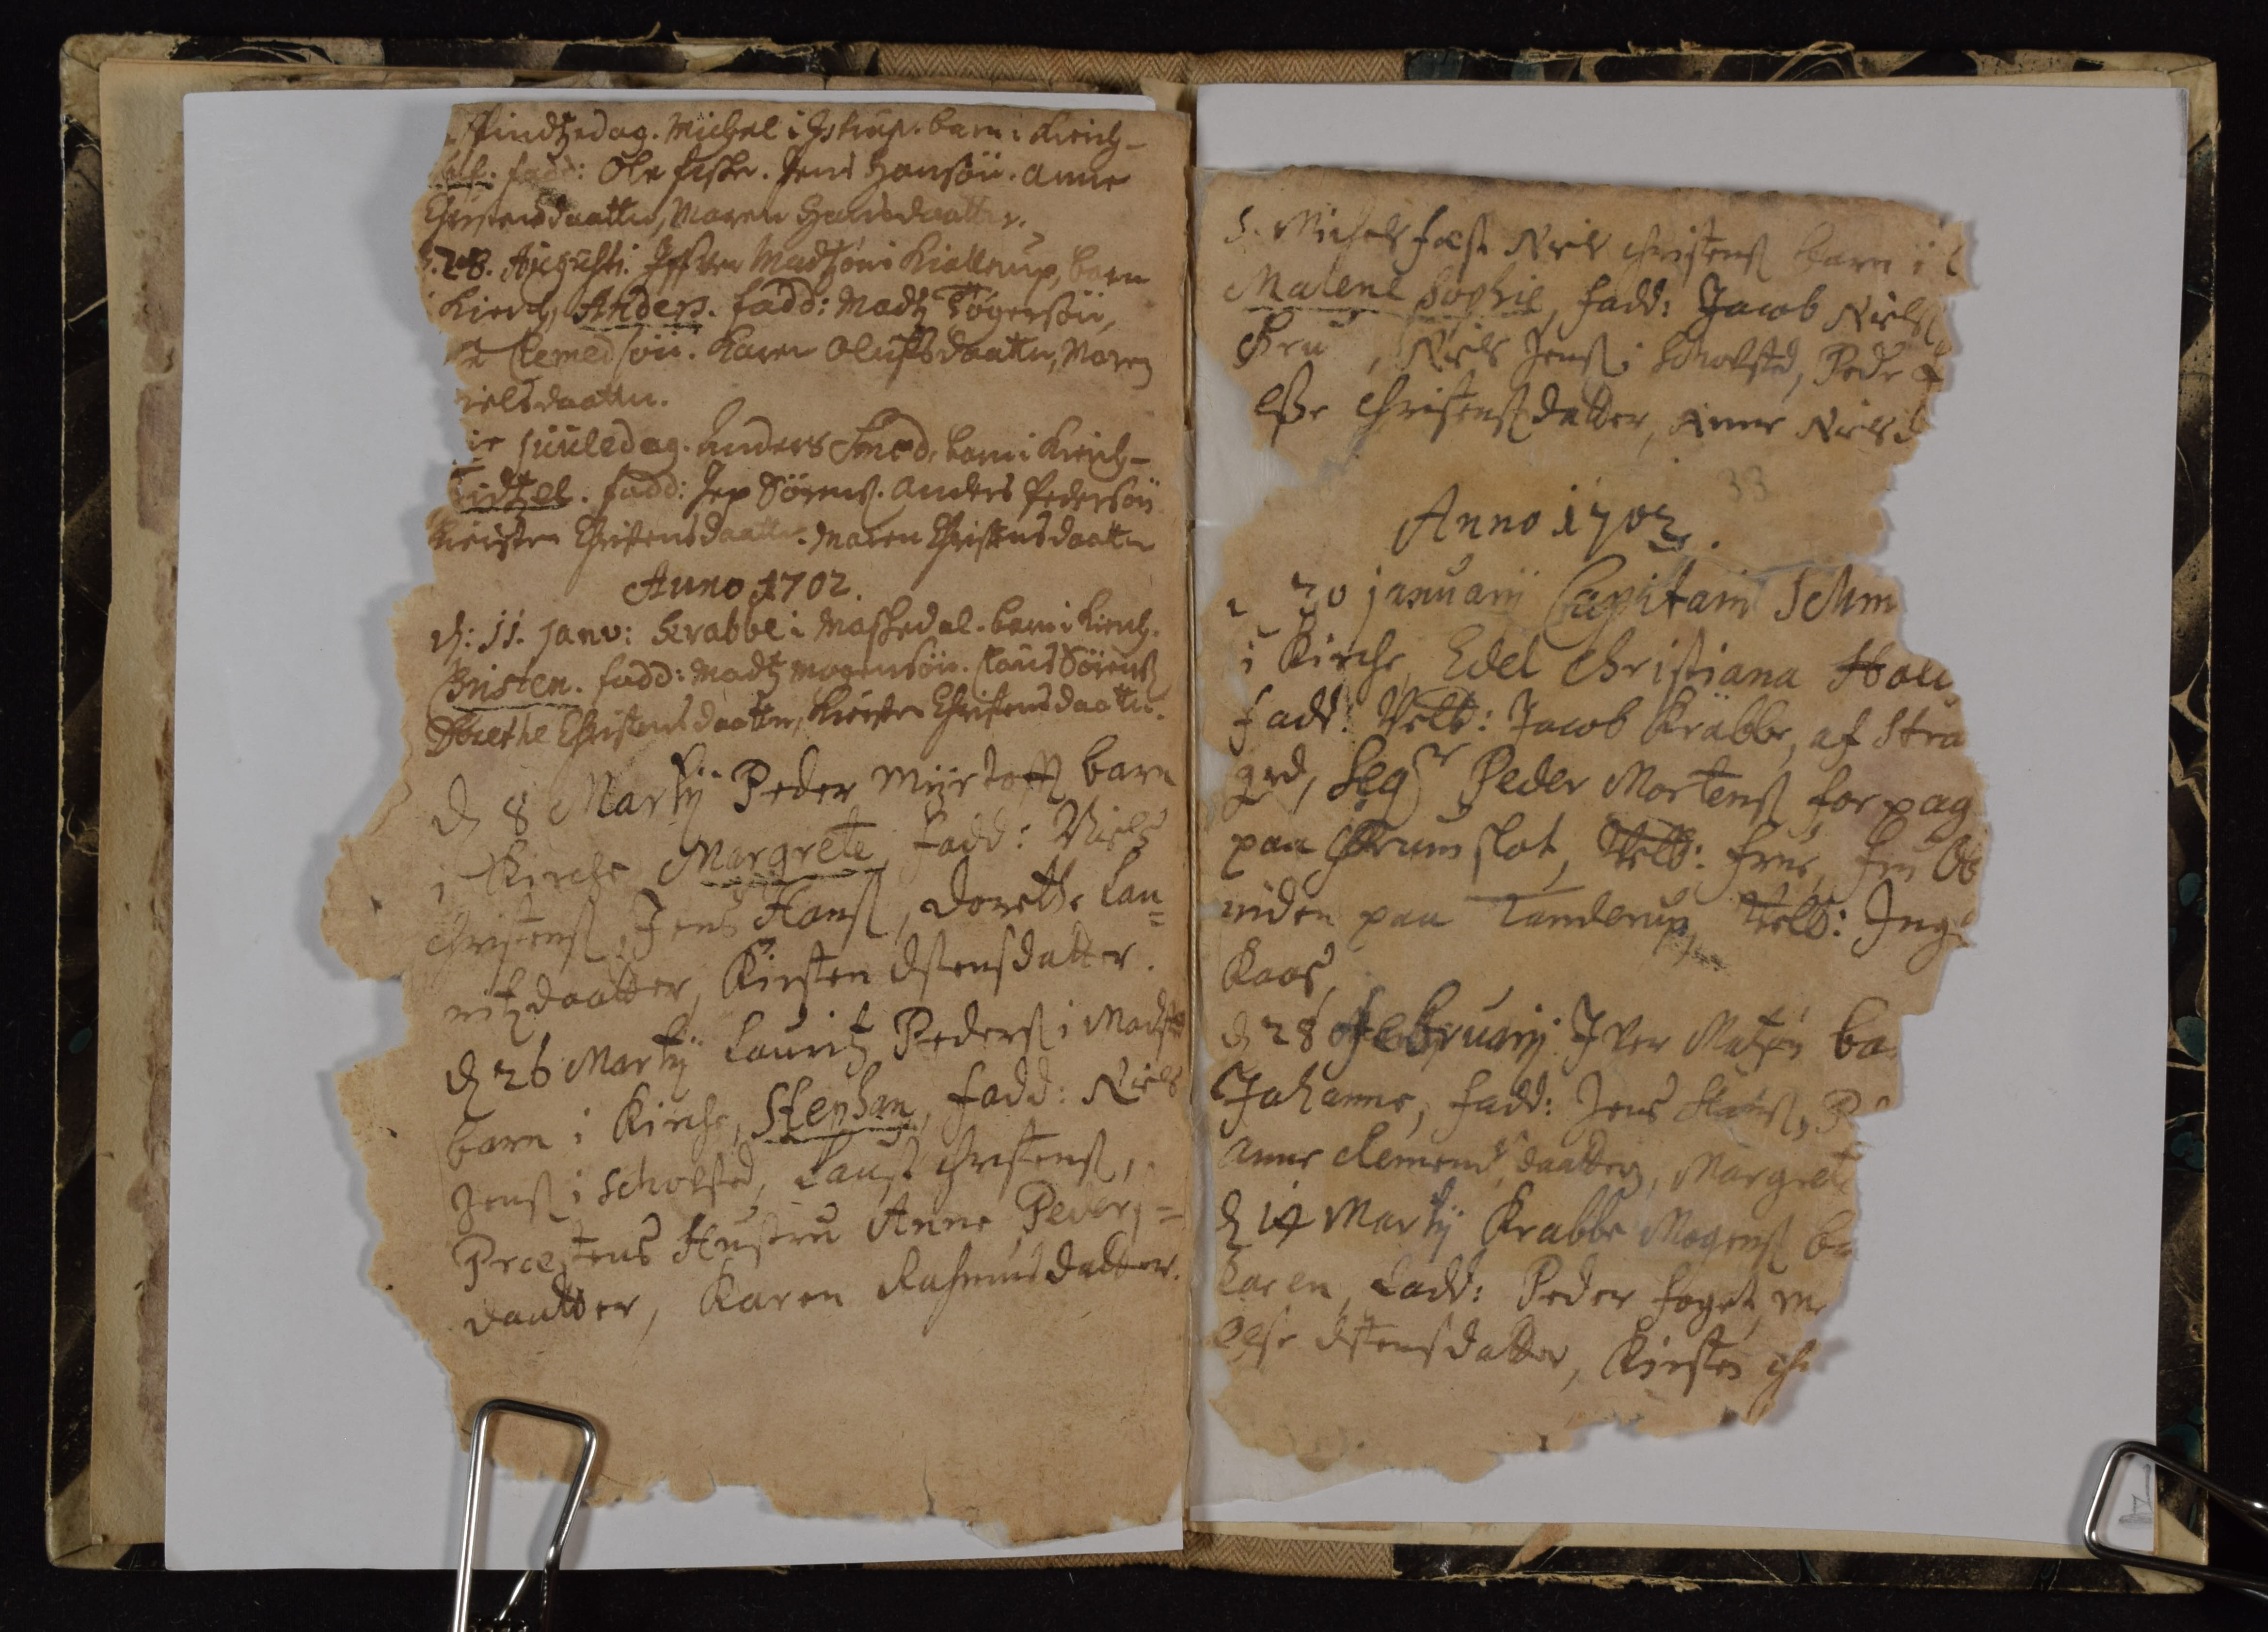Pindzedag Michel i Istrup. barn i Kirch
## fadd: Ole fisker. Jenst Hansøn. Anne
Chirstendaatter, Maren Hansdaatter.
28. Augusti Iffver Madtzøn Kiallrup, barn
Kirch, Anders. fadd: Madtz Tøgersøn,
## Clemedsøn. Karen Olufsdaater, Maren
Nielsdaatter.
Juuledag Anders Smed, barn i Krich
Zidtzel. fadd: Jep Sørens. Anders Pedersøn
Kiersten Christensdaater. Maren Christensdaater
Anno 1702.
: 11. Janv: ##abbe i Maskedal. barn i Kierch.
Christen. fadde Madtz Mogensøn. Claus Sørens
Dorethe Christensdaatter, Kiersten Christens datter.
d 8 Martij Peder Myetoff barn
i Kirche Margrete Fadd: Niels
Christens, Jens Hans, Dorethe Lau¬
ritzdaatter, Kirsten Ostensdatter.
d. 26 Martjj Lauritz Peders i Madst
barn i Kirche Sephen, Fadd: Niels
Jens i Schovsted, Laust Christens,
Præstens Hustru Anne Peders¬
daatter, Karen Rasmusdatter
S. Michels Fæst Niels Christens barn i
Malene Sophie, fadd Jacob Niels
Fru Niels Jens i Schovsted, Pedr
lse Christenssdatter, Anne Nielsd
Anno 1702
20 januarii Capitain Schm
i Kirche, Edel Christiana Ho## 
Fadd: Velb: Jacob Krabbe, af Stra
grd, Segr Peder Mortens forpag
paa Ørum slot, Velb: Frue Fru ##
inden paa Tanderup Velb: Ing
Kaas
d 28 Februarij. Iver Matzøn ba
Jahanne, Fadd: Jens Hans, ##
Anne Cemends daatter, Margrete
d 14 Martij Krabbe Mogens ba
Karen, Fadd: Peder foget m
Else ##stensdatter, Kirsten Ch
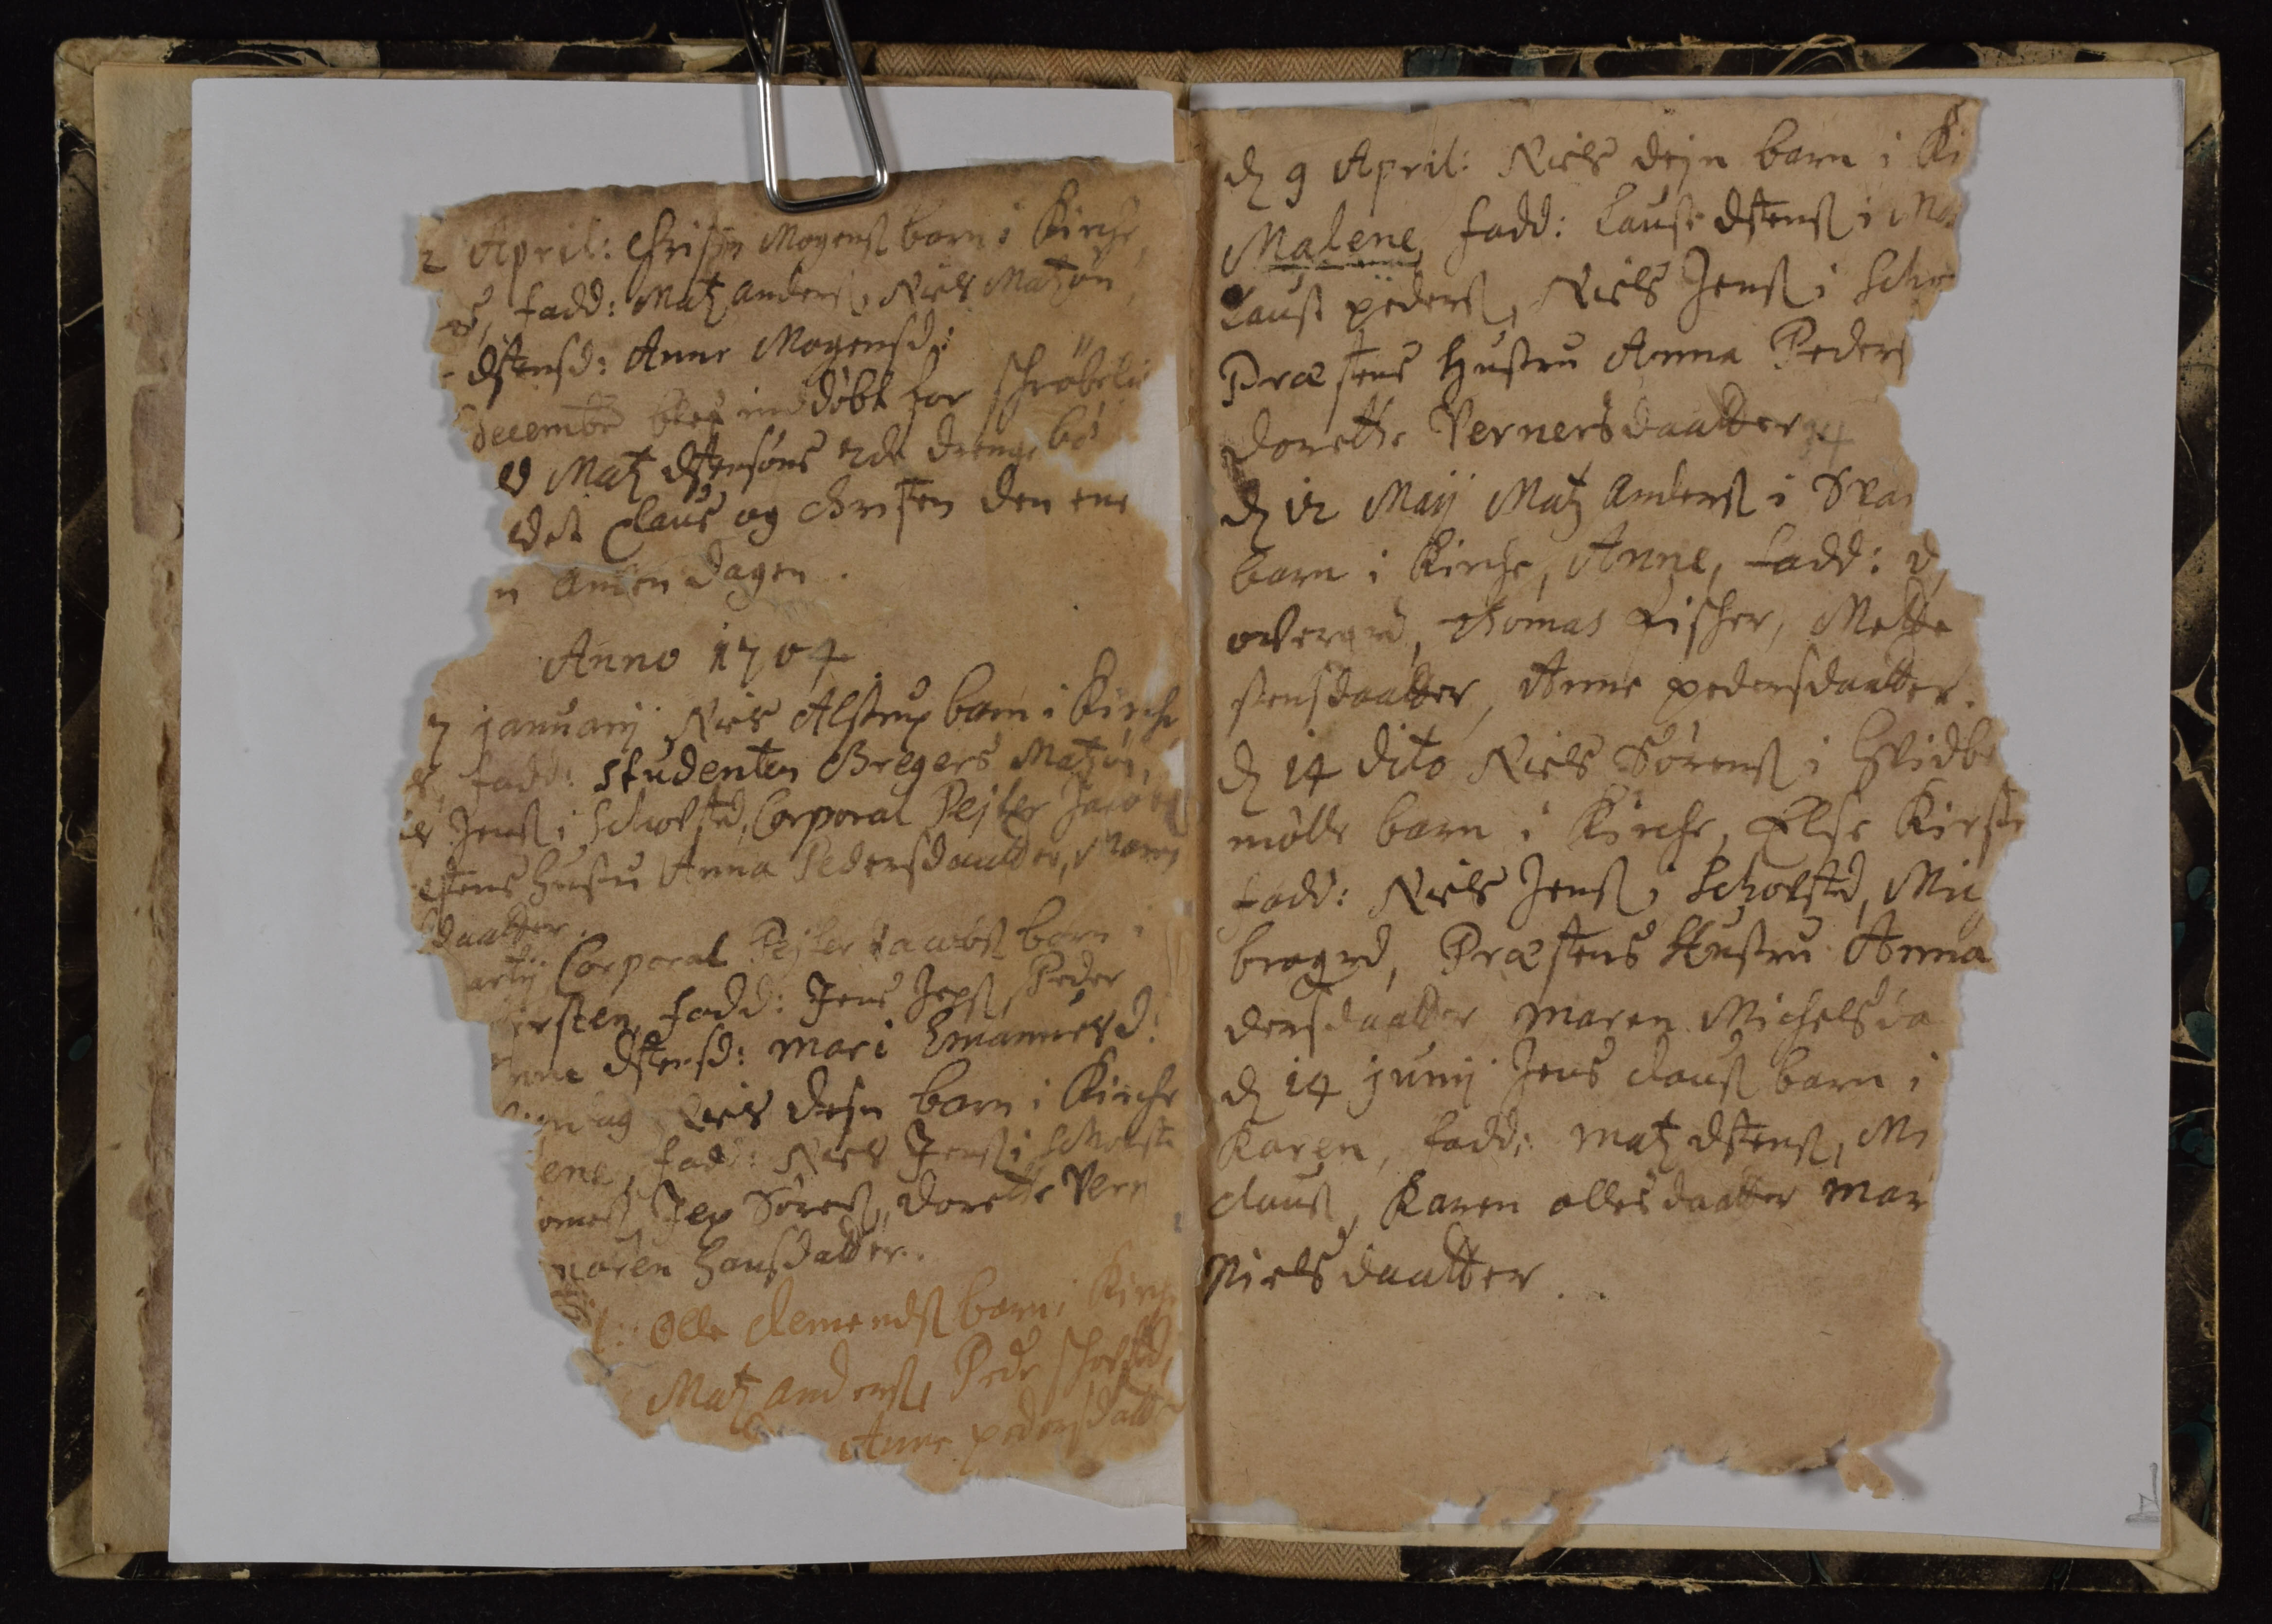2 April. Christen Mogens born i Kirche
s, Fadd: Matz Anders Niels Matzøn
##stensd: Anne Mogensd:
december blef inddøbt for shrøbeli
## Matz ##stensøns 2de drege bø
det Claus og Christen den en
Anden Dagen
Anno 1704
7 Januarij Niels Alstrup barn i Kirche
fadd: Studenten Gregers Matzøn,
Jens i Schovsted, Corporal Pejter Jacobs
stens Hustru Anna Pedersdaatter, Maren
daatter
##artij Corporal Pejter Jacobs born i
irsten, Fadd: Jens Jeps, Peder
nne ##stenbsd: mari Emanuelsd:
##dag Niels dejn barn i Kirche
ene, Fadd: Niels Jens i Schovste
omes Jep Sørens, Dorette Vern
aren Hansdatter.
Olle clemends barn i Kirch
Mats Anders, Pedr schovsted,
Anne pedersdatter
d 9 April: Niels dejn barn i Ki
Malene Fadd. Laust ##stens i M
Laust Peders, Niels Jens i Scho
Præstens Hustru Anna Peders
Dorethe Vernersdaatter
d 12 Maij Matz Anders i Sva
barn i Kirche Anne, Fadd: d
overgrd, Thomas Fisher, Mette
stensdaatter, Anne Pedersdaatter.
d 14 dito Niels Sørens i Hvidbe
mølle barn i Kirche, Else Kirst
Fadd: Niels Jens i Schovsted, Mic
brogrd; Præstens Hustru Anna
dersdaatter Maren Michelsda
d: 14 Junij Jens claus barn i
Karen, Fadd: Matz ##stens, Mi
Claus, Karen Ollesdaatter Mar
Nielsdaatter